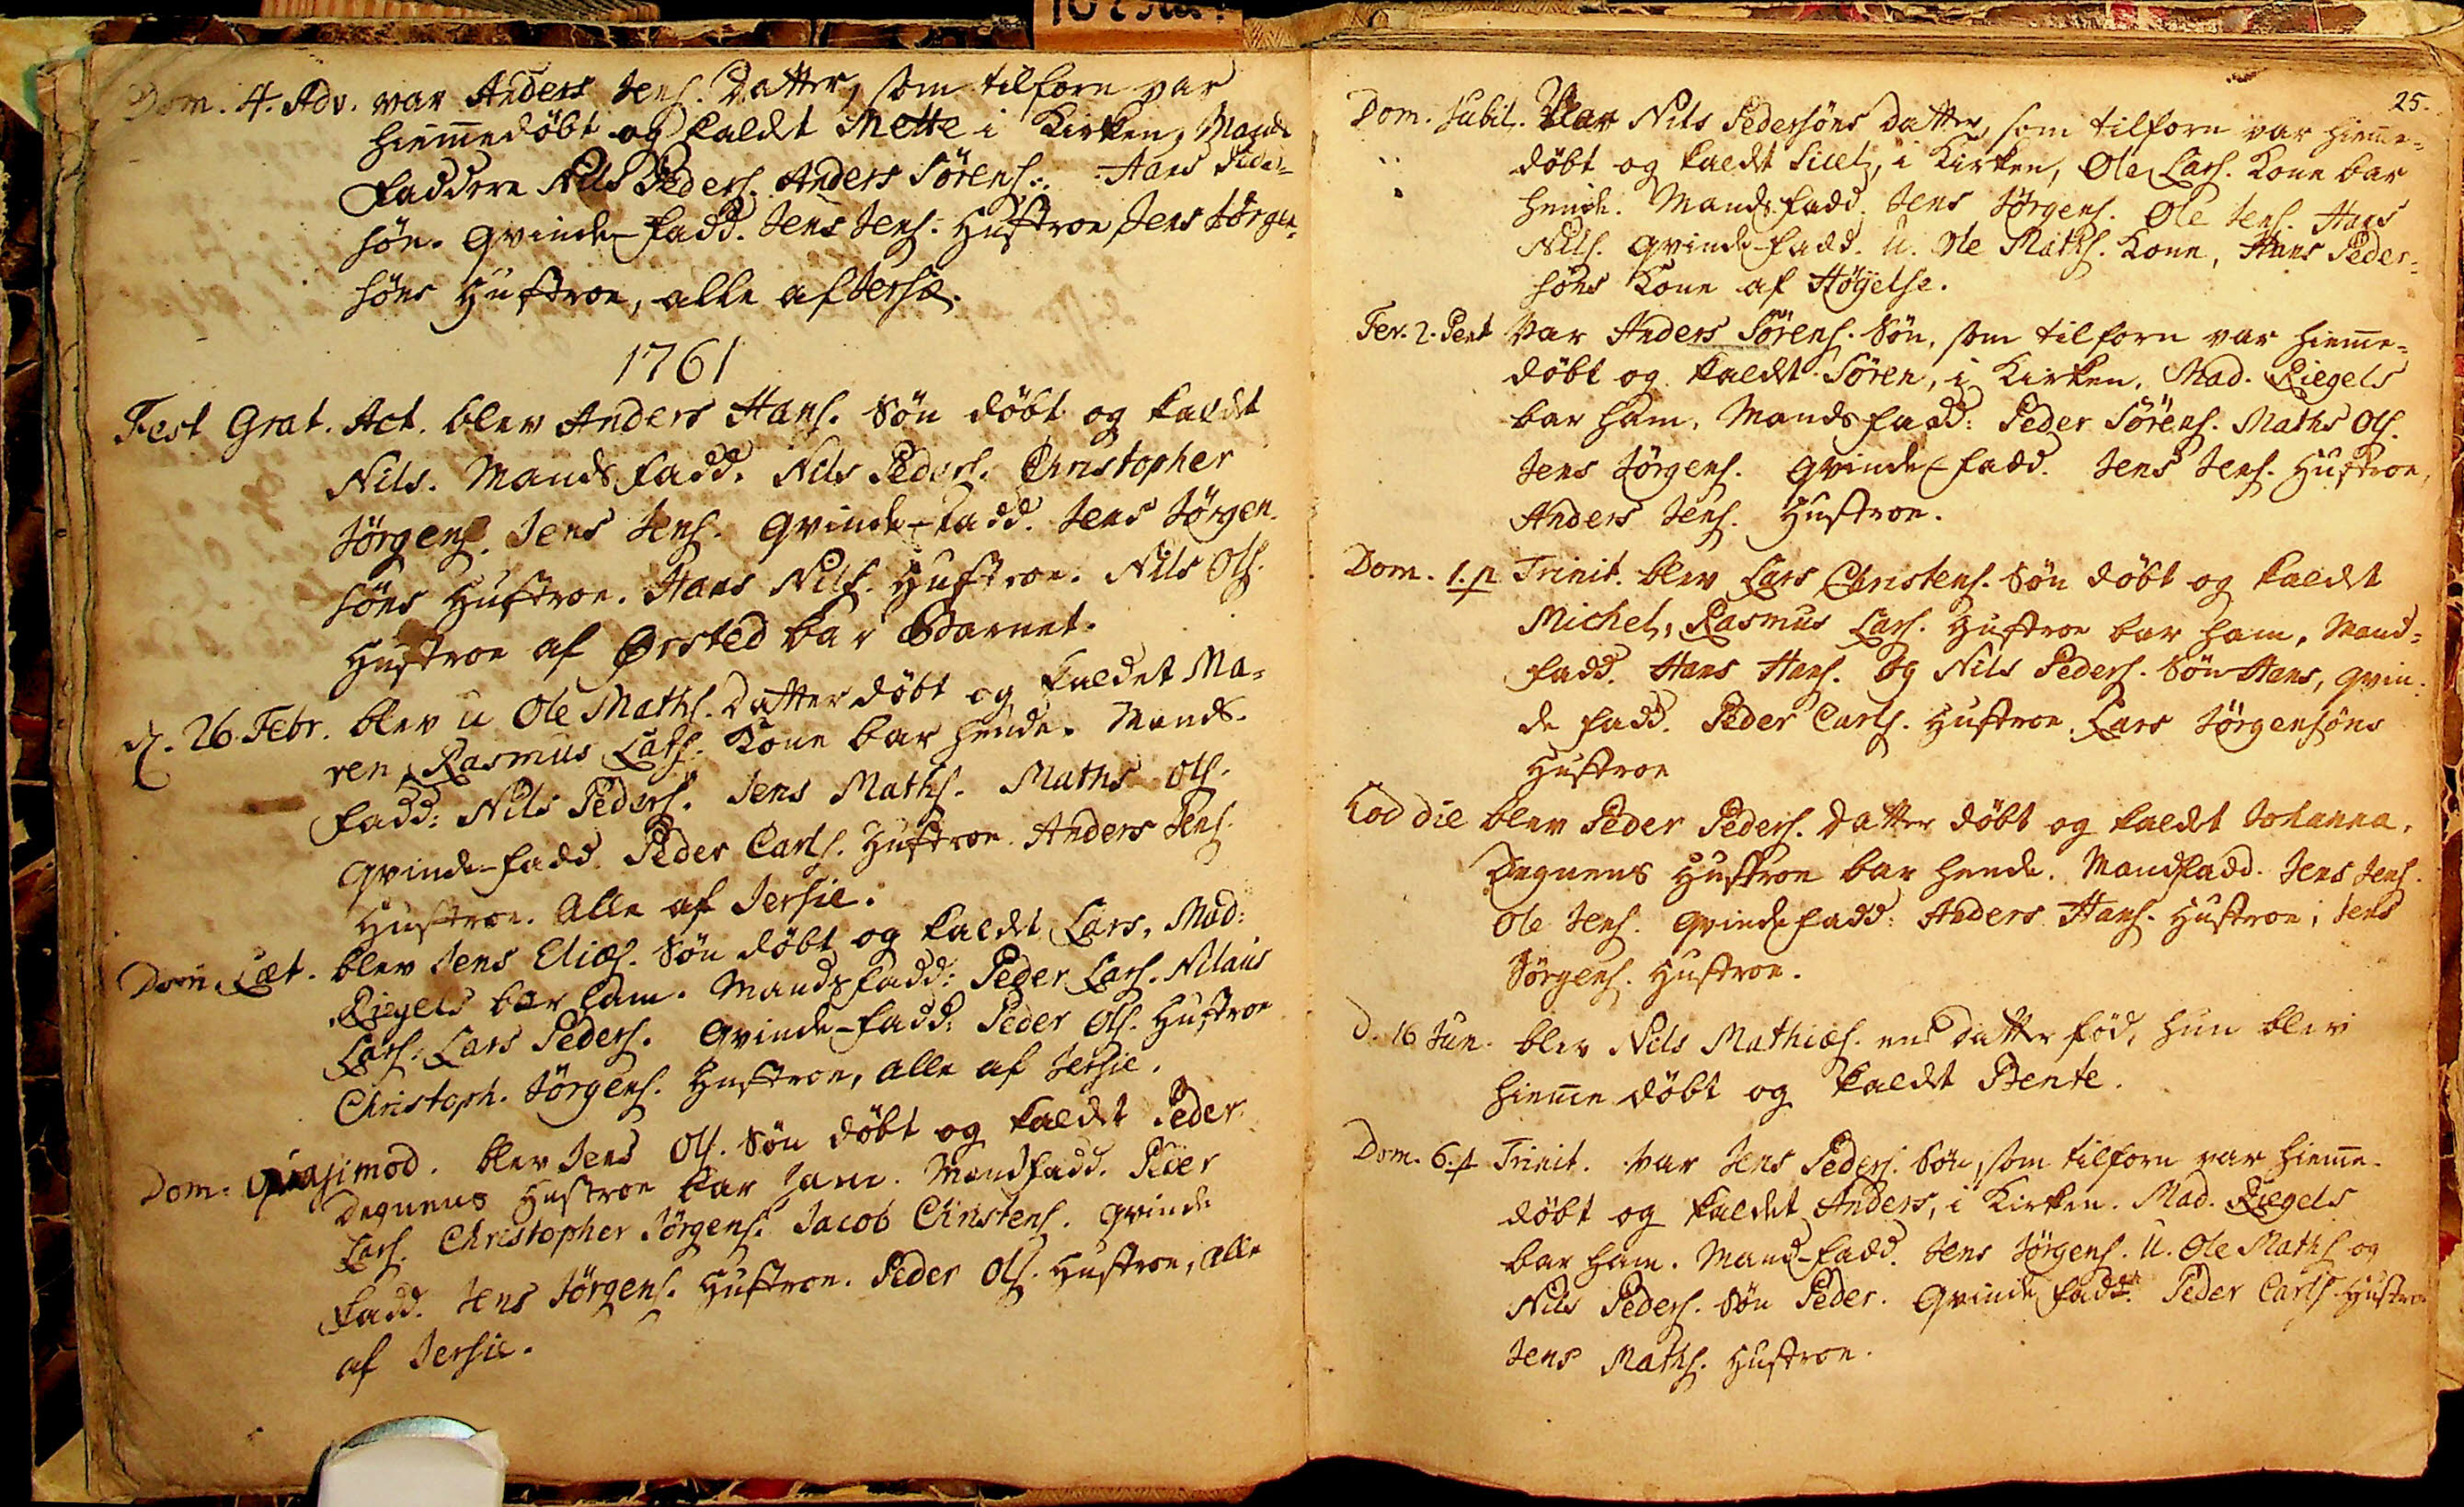Dom. 4. Adv. var Anders Jens. Datter, som tilforn var
hiemmedøbt og kaldt Mette i Kirken, Mands
Faddere Nils Peders. Anders Sørens:. Hans Peder¬
søn. Qvinde fadd. Jens Jens. Hustroe, Jens Jørgen¬
søns Hustroe, alle af Jersie.
1761
Fest Grat. Act. blev Anders Hans. Søn døbt og kaldt
Niels. Mands fadd. Nils Peders. Christopher
Jørgens. Jens Jens. Qvinde-fadd. Jens Jørgen¬
søns Hustroe. Hans Nils. Hustroe. Nils Ols.
Hustroe af Ørsted bar Barnet.
d. 26 Febr. blev u Ole Maths. Datter døbt og kaldet Ma¬
ren, Rasmus Lars. Kone bar hende. Mands¬
Fadd: Nils Peders. Jens Maths. Maths Ols.
Qvinde-fadd. Peder Carls. Hustroe. Anders Jens.
Hustroe. Alle af Jersie.
Dom. Læt. blev Jens Eliæs. Søn døbt og kaldt Lars, Mad:
Riegels bar ham. Mandsfadd: Peder Lars. Nilaus
Lars: Lars Peders. Qvinde-fadd: Peder Ols. Hustroe
Christopher Jørgens. Hustroe, alle af Jersie.
Dom Qvasimod. blev Jens Ols. Søn døbt og kaldt Peder
Degnens Hustroe bar ham. Mandfadd. Peder
Lars. Christopher Jørgens. Jacob Christens. qvinde
fadd. Jens Jørgens. Hustroe. Peder Ols. Hustroe, alle
af Jersie.
25.
Dom. Jubil. Var Nils Pedersøns Datter, som tilforn var hiemme¬
døbt og kaldt Sicel, i Kirken, Ole Lars. Kone bar
hende. Mands. fadd. Jens Jørgens. Ole Jens. Hans
Nils. Qvinde-fadd. U. Ole Maths. Kone, Hans Peder¬
søns Kone af Høyelse.
Fer. 2. Pent var Anders Sørens. Søn, som tilforn var hiemme¬
døbt og kaldt Søren, i Kirken. Mad. Riegels
bar ham. Mandsfadd: Peder Sørens. Maths Ols.
Jens Jørgens. Qvinde-fadd. Jens Jens. Hustroe,
Anders Jens. Hustroe.
Dom. 1. p Trinit blev Lars Christens. Søn døbt og kaldt
Michel, Rasmus Lars. Hustroe bar ham. Mand¬
fadd. Hans Hans. og Nils Peders. Søn Hans, Qvin¬
de fadd. Peder Carls. Hustroe. Lars Jørgensøns
Hustroe
Eod die blev Peder Peders. Datter døbt og kaldt Johanna.
Degnens Hustroe bar hende. Mandfadd. Jens Jens.
Ole Jens. Qvindefadd: Anders Hans. Hustroe, Jens
Jørgens. Hustroe.
D. 16 Jun. blev Nils Mathiæs. en Datter fød, hun blev
hiemmedøbt og kaldt Bente.
Dom. 6. p Trinit. var Jens Peders. Søn, som tilforn var hiemme¬
døbt og kaldet Anders, i Kirken. Mad Riegels
bar ham. Mand-fadd. Jens Jørgens. U. Ole Maths og
Nils Peders. Søn Peder. Qvinde fader Peder Carls. Hustroe
Jens Maths. Hustroe.
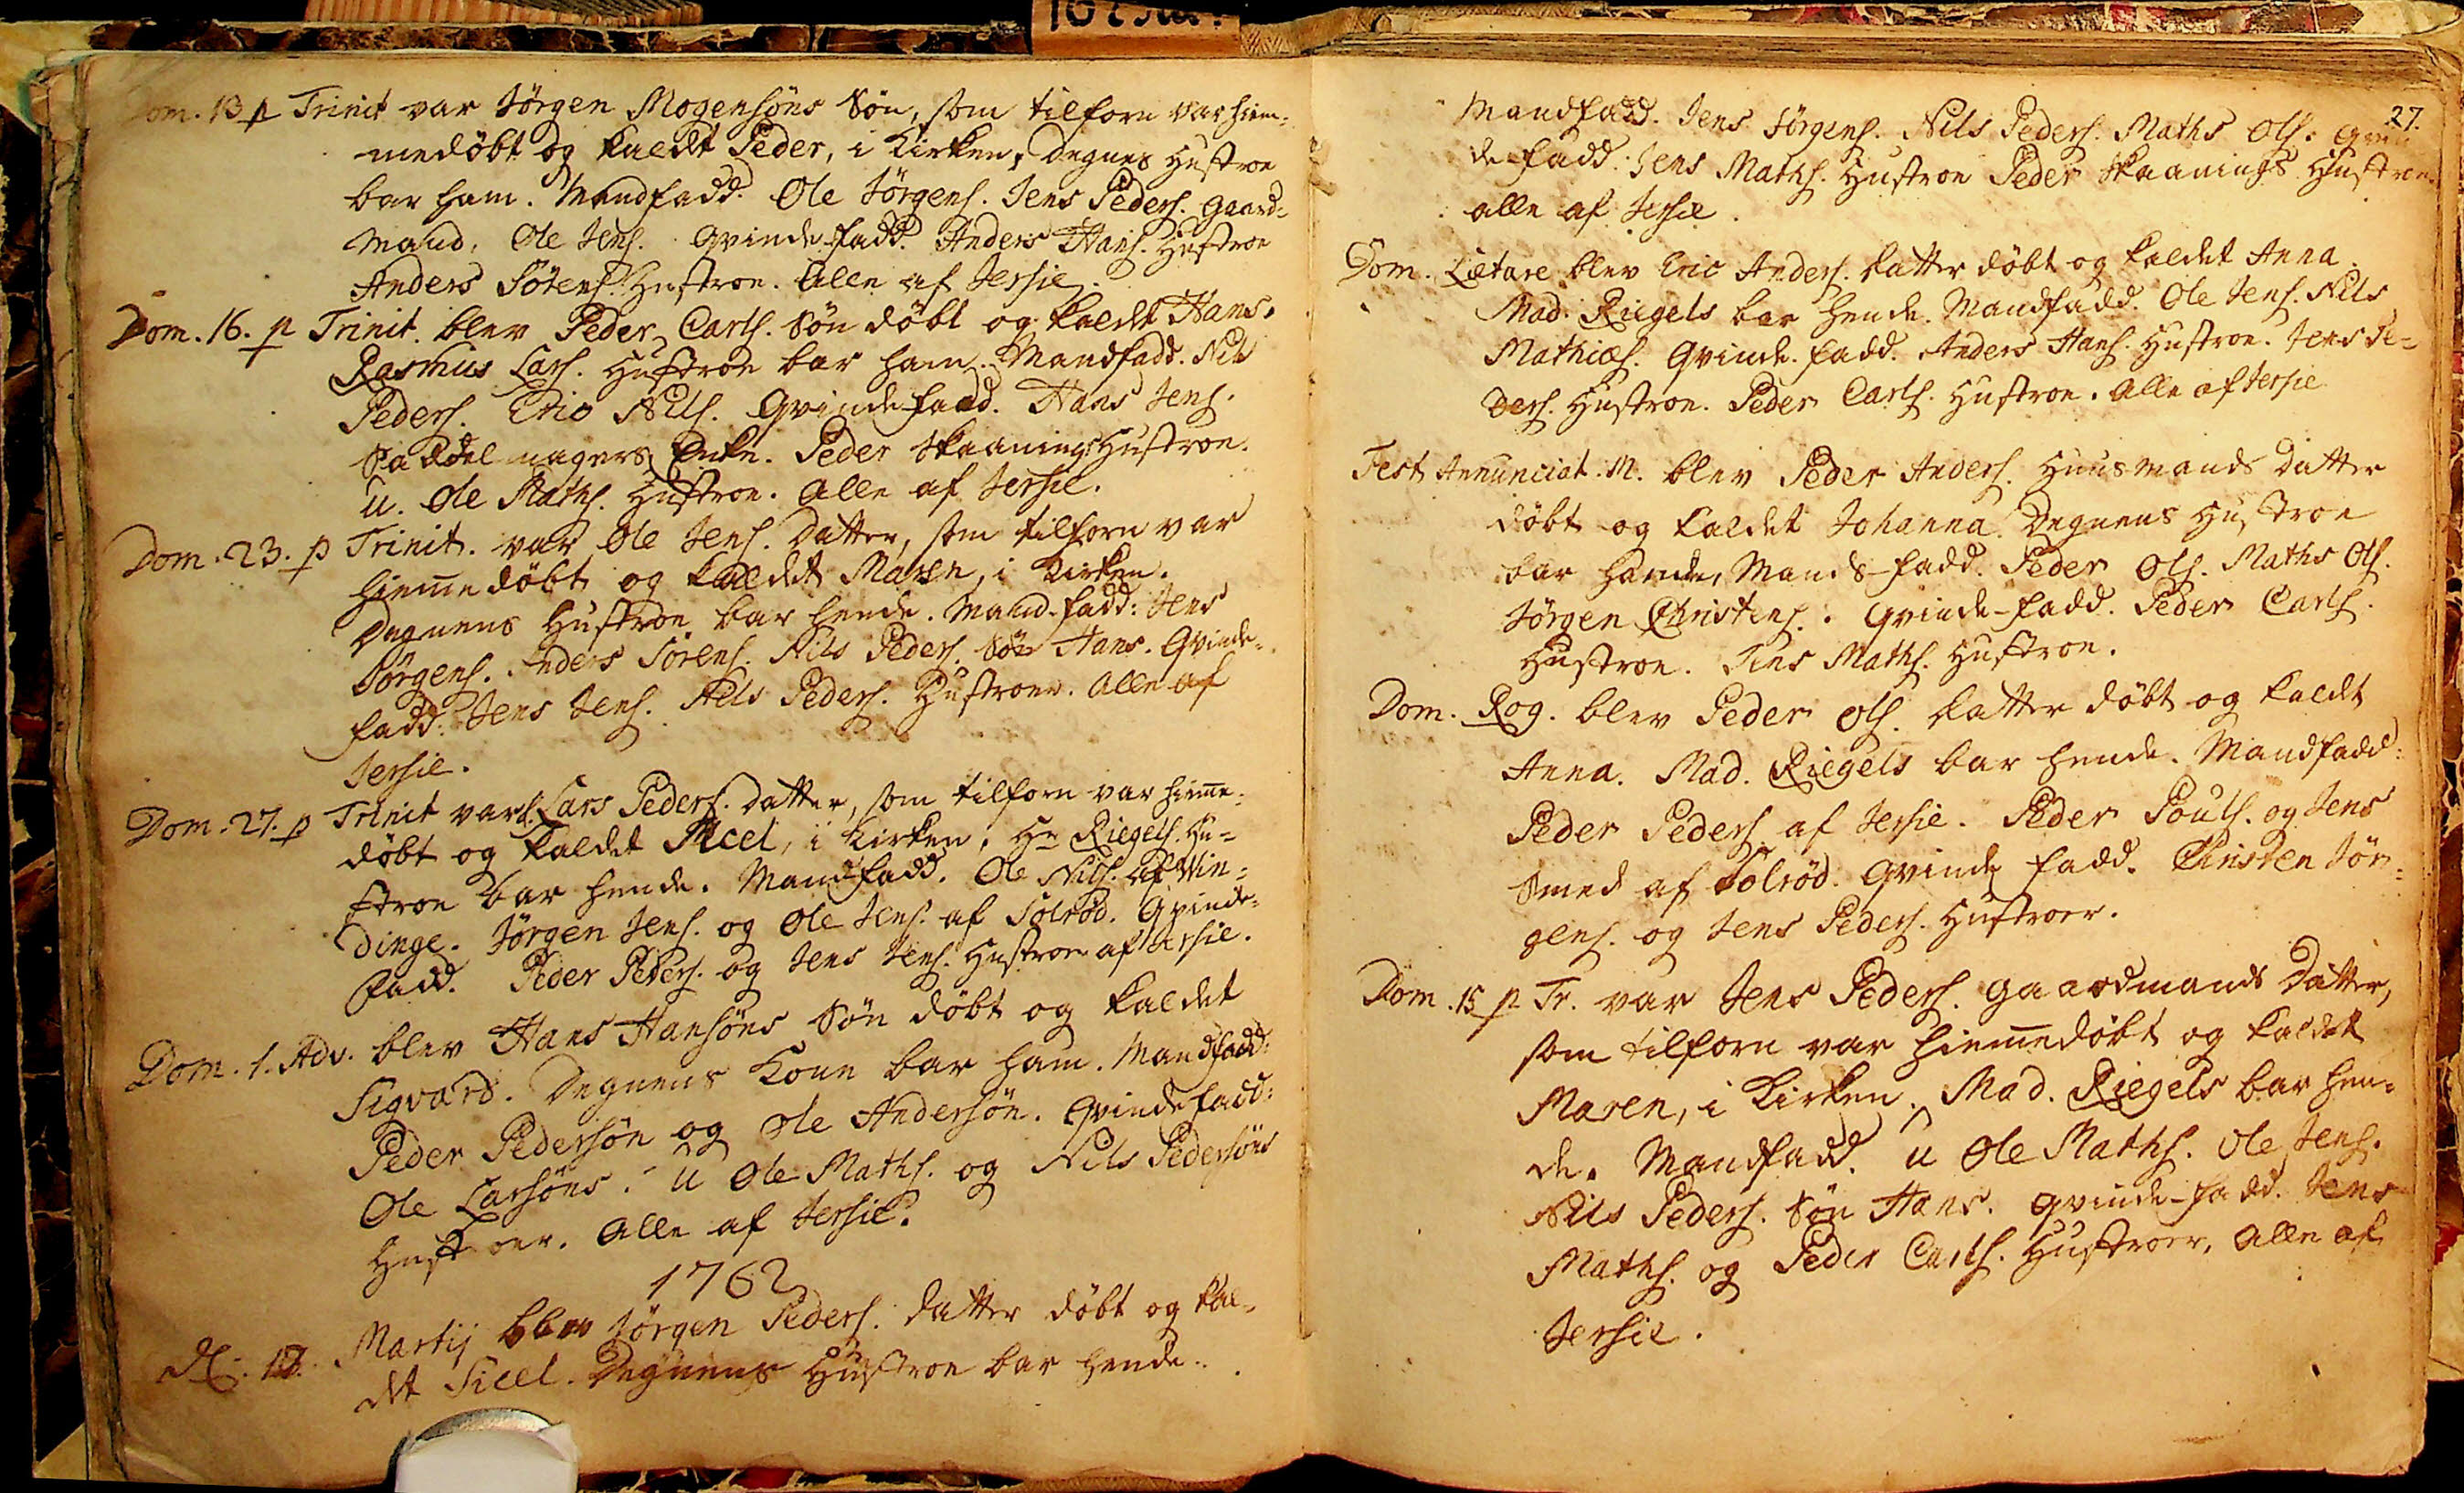Dom. 13 p Trinit var Jørgen Mogensøns Søn, som tilforn var hiem¬
medøbt og kaldt Peder, i Kirken, Degnens Hustroe
bar ham. Mandfadd. Ole Jørgens. Jens Peders. Gaard¬
mand, Ole Jens. Qvinde-fadd. Anders Hans. Hustroe
Anders Sørens. Hustroe. Alle af Jersie.
Dom. 16. p Trinit. blev Peder Carls. Søn døbt og kaldt Hans.
Rasmus Lars. Hustroe bar ham. Mandfadd. Nils
Peders. Eric Nils. Qvinde-fadd. Hans Jens.
Saddelmagers Enke. Peder Skaanings Hustroe.
U. Ole Maths. Hustroe. Alle af Jersie.
Dom. 23. p Trinit. var Ole Jens. Datter, som tilforn var
hiemmedøbt og kaldet Maren, i Kirken.
Degnens Hustroe bar hende. Mand-fadd: Jens
Jørgens. Anders Sørens. Nils Peders. Søn Hans. Qvinde¬
fadd: Jens Jens. Nils Peders. Hustroer. Alle af 
Jersie.
Dom. 27.p Trinit var Lars Peders. Datter, som tilforn var hiemme¬
døbt og kaldet Sicel, i Kirken, Hr Riegels. Hu¬
stroe bar hende. Mand-fadd. Ole Nils. af Win¬
dinge. Jørgen Jens. og Ole Jens. af Solrød. Qvinde¬
fadd. Peder Peders. og Jens Jens. Hustroe af Jersie.
Dom. 1. Adv. blev Hans Hansøns Søn døbt og kaldet
Sigvard. Degnens Kone bar ham. Mandfadd:
Peder Pedersøn og Ole Andersøn. Qvindefadd:
Ole Larsøns. U Ole Maths. og Nils Pedersøns
Hustroer. Alle af Jersie.
1762
d: 19. Martii blev Jørgen Peders. datter døbt og kal¬
dt Sicel. Degnens Hustroe bar hende.
27.
Mandfadd. Jens Jørgens. Nils Peders. Maths Ols. Qvin
de-fadd: Jens Maths. Hustroe Peder Skaanings Hustroe
alle af Jersie.
Dom. Lætare blev Eric Anders. Datter døbt og kaldet Anna.
Mad. Riegels bar hende. Mandfadd. Ole Jens. Nils
Mathiæs. Qvinde.fadd. Anders Hans. Hustroe. Jens Pe¬
ders. Hustroe. Peder Carls. Hustroe. Alle af Jersie.
Fest Annunciat. M. blev Peder Anders. Huusmands Datter
døbt og kaldet Johanna. Degnens Hustroe
bar hende, Mands-fadd. Peder Ols. Maths Ols.
Jørgen Christens. Qvinde-fadd. Peder Carls.
Hustroe. Jens Maths. Hustroe.
Dom. Rog. blev Peder Ols. Datter døbt og kaldt
Anna. Mad. Riegels bar hende. Mandfadd:
Peder Peders. af Jersie. Peder Pouls. og Jens
Smed af Solrød. Qvinde fadd. Christen Jør¬
gens. og Jens Peders. Hustroer.
Dom. 15 p Tr. var Jens Peders. Gaardmands Datter,
som tilforn var hiemmedøbt og kaldet
Maren, i Kirken. Mad. Riegels bar hen¬
de. Mandfadd. U Ole Maths. Ole Jens.
Nils Peders. Søn Hans. Qvinde-fadd. Jens
Maths. og Peder Carls. Hustroer, alle af
Jersie.